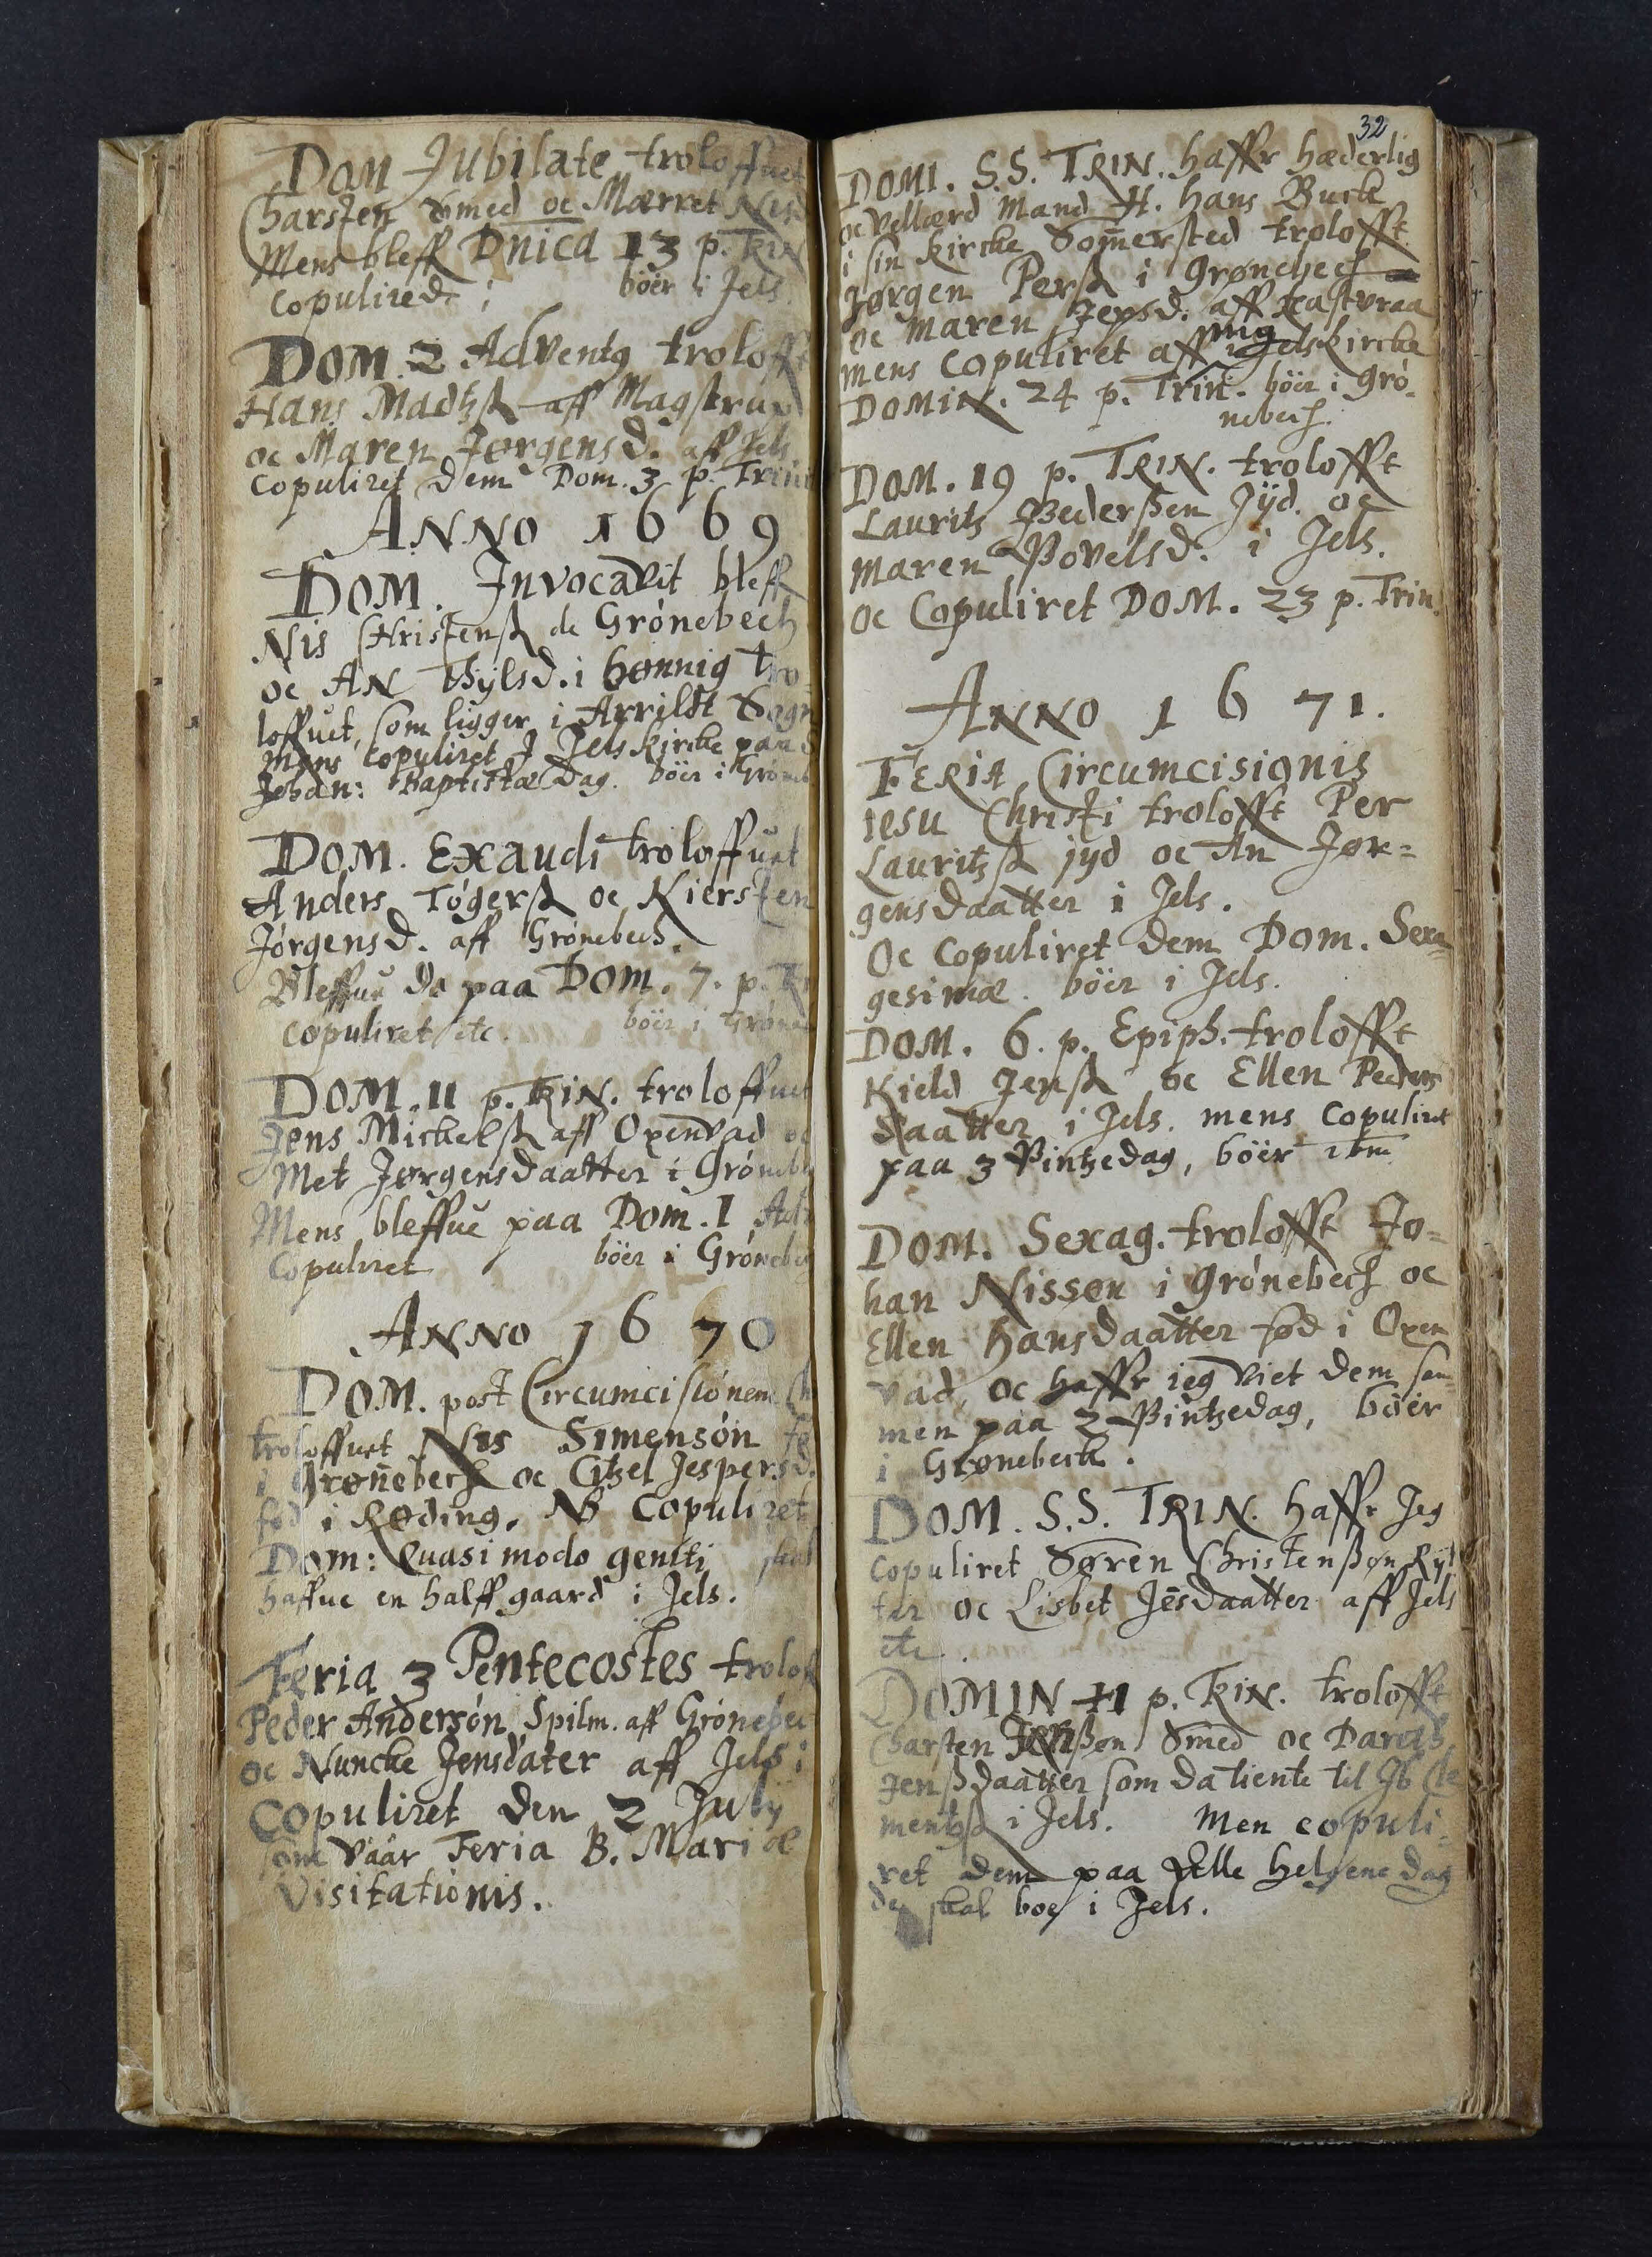DOM Jubilate troloffuet
Charsten Smed oc Marret Nisd.
Mens bleff Dnica 13 p. TRIN.
copulirede: boer i Jels.
DOM 2. Adventq trolofft
Hans Madtzs aff Magstrup
oc Maren Jørgensd. aff Jels.
Copuliret dem Dom. 3 p. Trinit
ANNO 1669
DOM. Invocavit bleff
Nis Christens de Grønnebech
oc An Thijlsd. I Hønnig tro¬
loffuet, som ligger i Arrildt Sogn
mens copuliret J Jels Kircke paa S
Johan: Baptista Dag. boer i Grøneb
DOM. Exaudi troloffuet
Anders Tøgers oc Kiersten
Jørgensd. aff Grønebech.
Bleffue de paa Dom. 7. p. Tr
copuliret etc. boer i Grøneb
DOM. II p. TRIN. troloffuet
Jens Mickels aff Oxenvad oc
Met Jørgens Daatter i Grønbe
Mens bleffue paa Dom. I Ad 
copuliret.
boer i Grønebe 
ANNO 1670
DOM. post Circumisionen Ch 
troloffuet NIS Simensøn
i Grønnebech oc Citzel Jespersd.
Fød i Røding. NB copuliret
Dom: Cuasi modo geniti skal
haffue en halff gaard i Jels.
Feria 3 Pentecostes trolof 
Peder Andersøn Spilm. aff Grønebec 
oc Nuncke Jensdater aff Jels:
copuliret den 2 Julij
som vaar Feria B. Maria
Visitationis.
32
DOMI. S.S. TRIN. Haffe Hæderlig
oc Vellærd Mand H. Hans Buck
i sin Kircke Sommersted trolofft
Jørgen Pers i Grønnebech
oc Maren Jepsd. aff Kastvraa
mig
mens copuliret aff i Jels Kircke
DOMIN. 24 p. Trin. boer i Grø¬
nebech.
DOM. 19 p. TRIN. trolofft
Lauritz Pederssen Jyd oc
Maren Povelsd. i Jels
oc copuliret DOM. 23 p. Trin.
ANNO 1671.
FERIA Circumcignis
iesu Christi trolofft Per
Lauritzs jyd oc An Jør¬
gens Daatter i Jels.
Oc Copuliret dem Dom. Sexa¬
gesima boer I Jels.
DOM. 6. P. Epiph. trolofft
Kield Jens oc Ellen Peders
daatter i Jels. Mens copuliret
paa 3 Pintzesag , boer ibm
DOM Sexag. trolofft Jo¬
han Nissen i Grønebech oc
Ellen Hansdaatter fød i Oxen
vad oc haffe ieg viet dem sam¬
men paa 2. Pintzedag, boer
i Grønebeck.
Dom S.S. TrRIN. haffe Jeg
copuliret Søren Christenssøn Ryt
ter oc Lisbet Jesdaatter aff Jels
etc.
DOMIN † 1 p. TRIN. trolofft
Charsten Jenssøn Smed oc Daras##
Jenssdaatter som da tiente til Ib Cle
mentzs i Jels. Men copuli¬
ret dem paa Alle Helgens Dag
de skal boe i Jels.
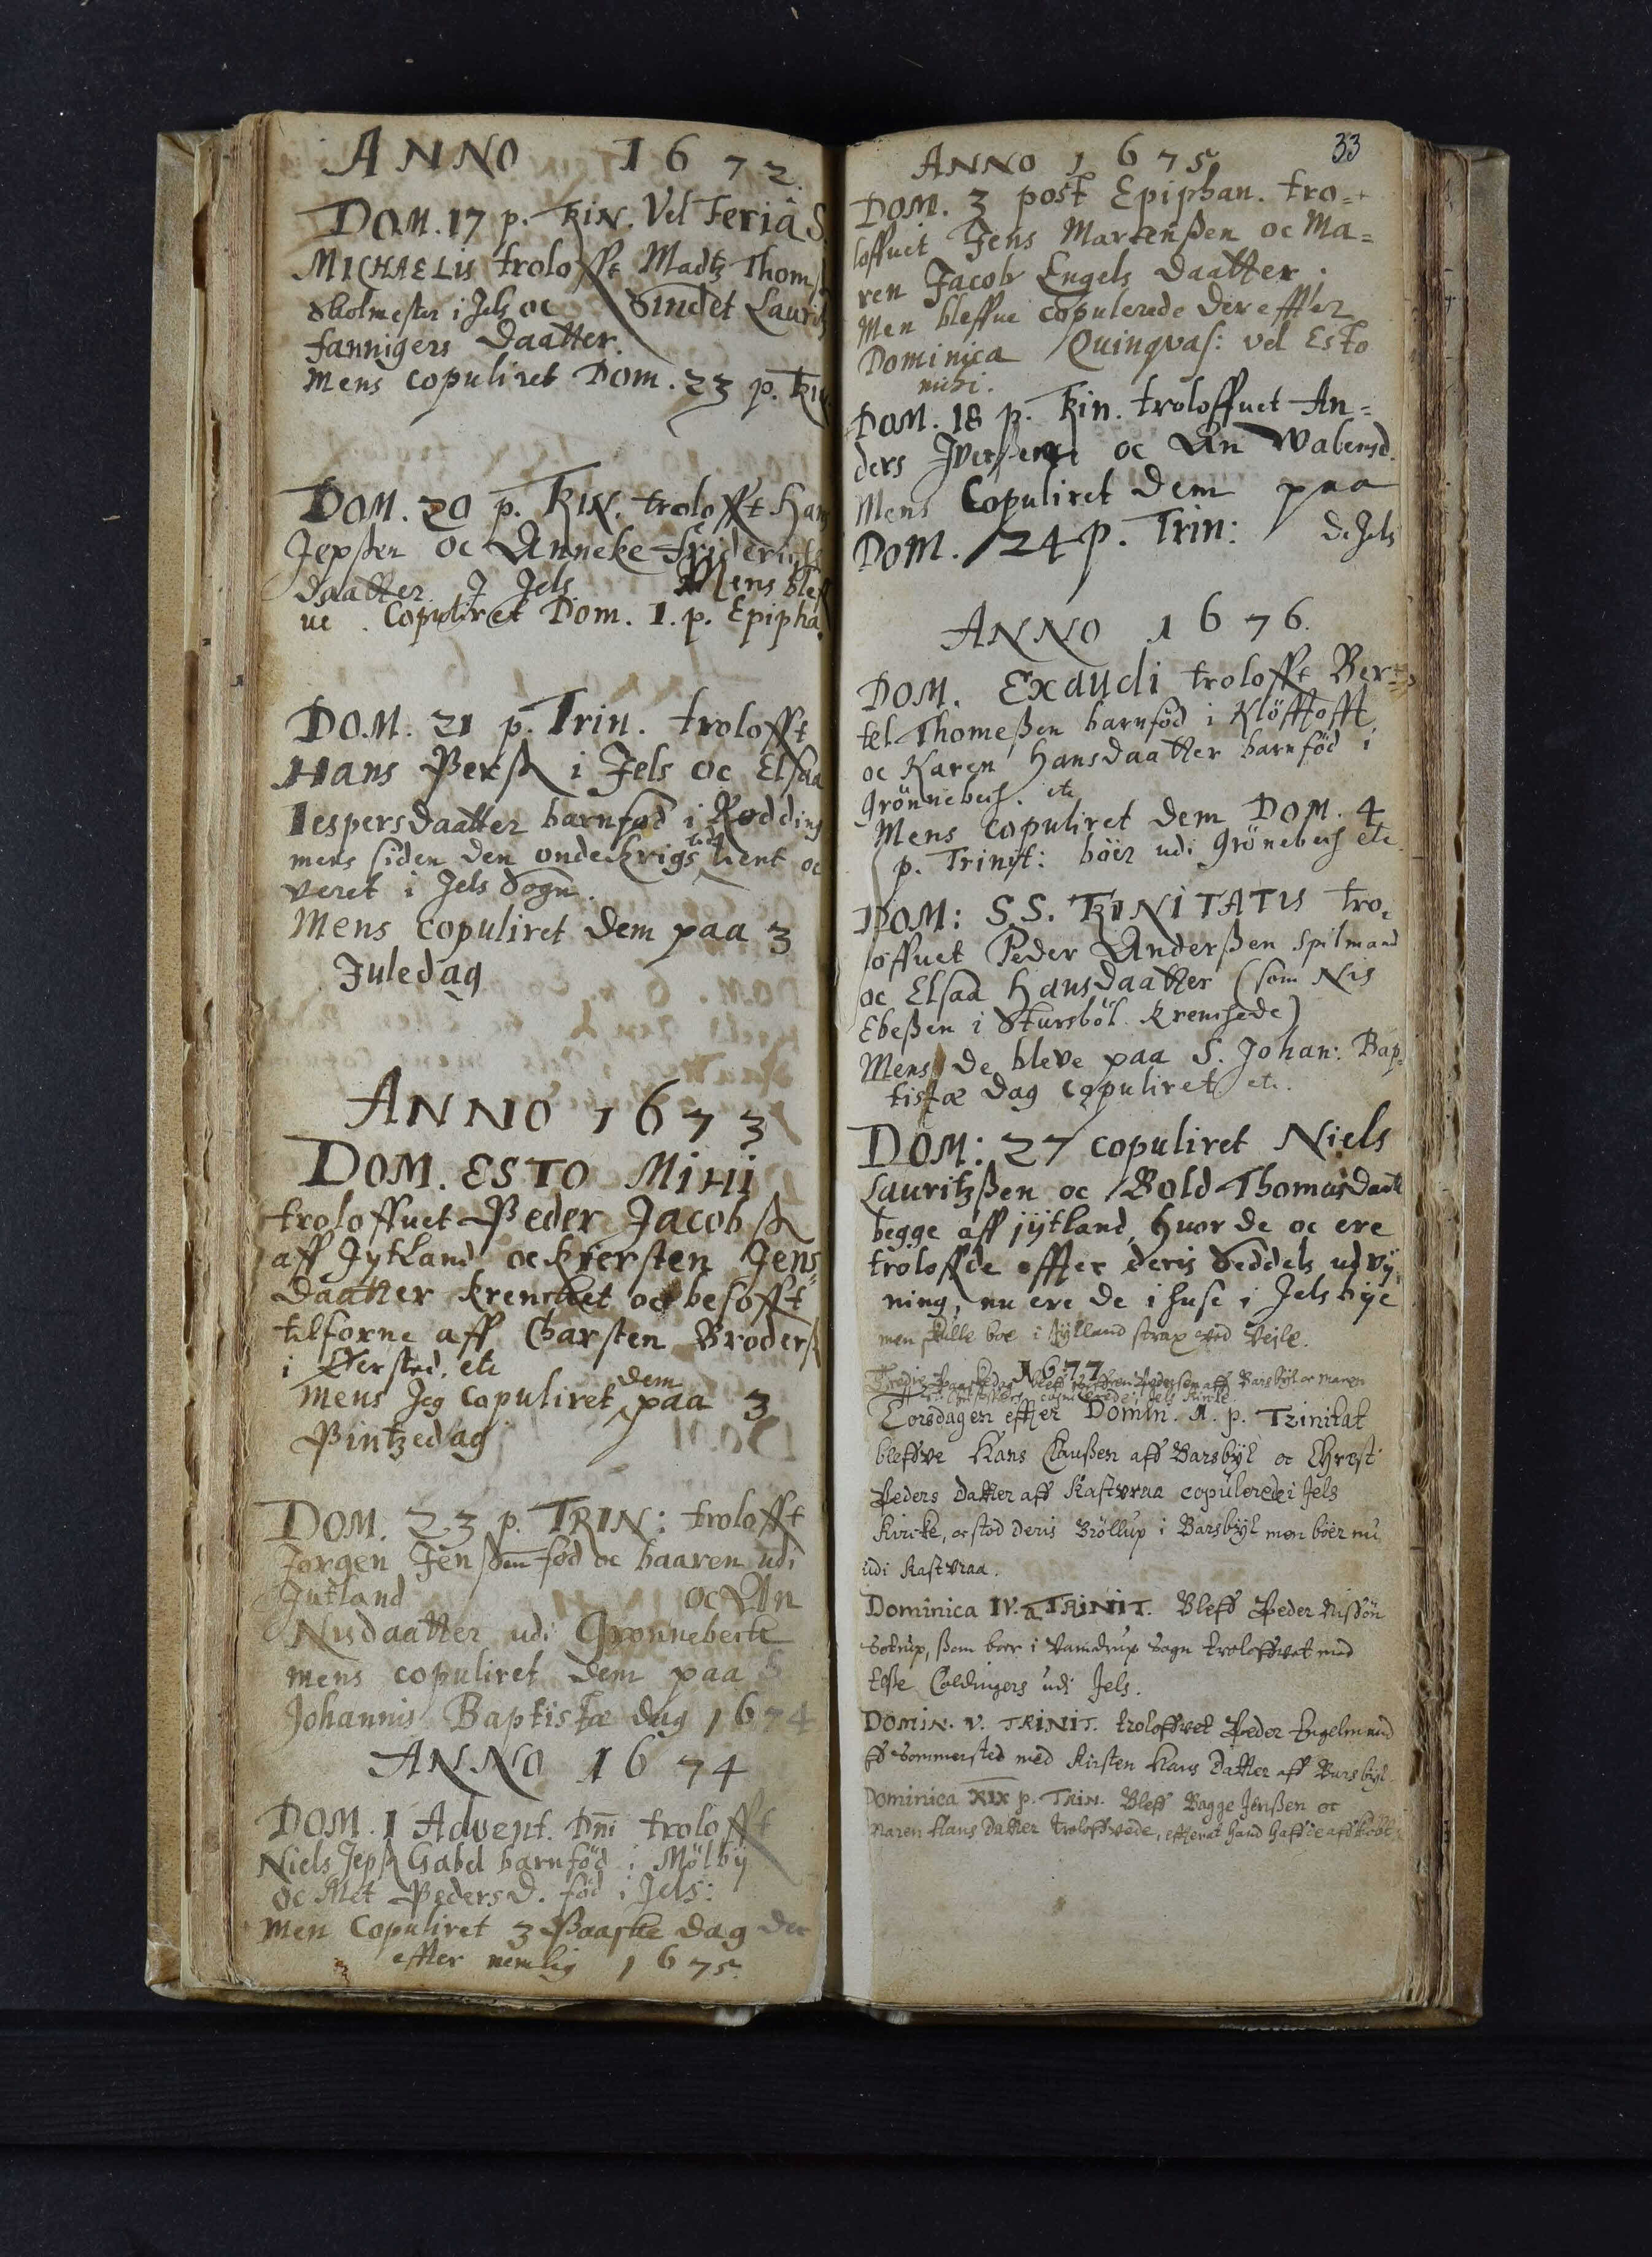ANNO 1672.
DOM. 17 p. TRIN. Vel Feria S
Michaelis trolofft Madtz Thoms 
Skolmester i Jels oc Sindet Lauritz
Fannigers Daatter.
Mens copuliret Dom. 23 p. Trin
DOM. 20 p. TRIN. trolofft Hans
Jepsen oc Anneke Friderichs
Daatter J Jels. Mens bleff
ue copuliret Dom. 1. p. Epipha
DOM. 21 p. Trin. trolofft
Hans Pers i Jels oc Elsaa
Iespers Daatter barnfød i Rødding
tid
mens siden den onde Krigs tient oc 
veret i Jels Sogn
Mens copuliret dem paa 3
Juledag
ANNO 1673.
DOM. ESTO MIHI
troloffuet Peder Jacobs
aff Jylland oc Kiersten Jens¬
Daatter krencket oc besofft
tilforne aff Charsten Broders
i Øersted etc.
dem
Mens Jeg copuliret paa 3
Pintzedag
DOM. 23 p. TRIN: trolofft
Jørgen Jensen fød oc baaren udi
Jutland
oc An
Nisdaatter udi Grønnebeck
mens copuliret dem paa S.
Johannis Baptista dag 1674
ANNO 1674
DOM. I Advent Dni trolofft
Niels Jeps Gabel barnfød i Mølby
oc Met Pedersd. fød i Jels:
men copuliret 3 Paaske Dag der
effter nemlig 1675.
33
ANNO 1675
DOM. 3 post Epiphan. Tro¬
loffuit Jens Martensen oc Ma¬
ren Jacob Engels Daatter.
Men bleffue copulerede der effter
Dominica Cuingvas: vel Esto
nuhi.
DOM. 18 p. TRIN. troloffuet An¬
ders Iversen oc An Wabensd.
Mens copuliret dem paa
Dom. 24 p. Trin: de Jels.
ANNO 1676
DOM. Exaudi trolofft Ber¬
tel Thomesen barnfød i Kløfftofft
oc Karen Hansdaatter barnfød i
Grønnebeck. etc.
Mens copuliret dem DOM. 4
p. Trinit: Boer udi Grønnebeck etc.
DOM: S. S. TRINITATIS tro¬
loffuet Peder Andersen Spilmand 
oc Elsaa Hansdaatter (som Nis 
Ebesen i Stursbøl krenchede)
Mens de bleve paa S. Johan: Bap¬
tista Dag copuliret etc.
DOM: 27 copuliret Niels
Lauritzsen oc Bold Thomasdaatt
begge aff Jytland, huor de oc ere
troloffde ##ffer deris Seddels udvy¬
ning, nu ere de i huse i Jels bye
men skulle boe i Jylland strax ved Vejle.
1677
Tredje Paaskedag bleff ## Pedersen aff Barsbyl oc Maren
S## Christoffers copulerede i Jels Kircke.
Torsdagen effter Domin. 1. p. Trinitat
bleffve Hans Clausen aff Barsbyl oc Chrest
Peders Datter aff Kastvraa copulerede i Jels
Kircke, oc stod deris Brøllup i Barsbyl men boer nu
udi Kastvraa.
Dominica IV. a. TRINIT. Bleff Peder Nissøn
Sotrup, som boer i Vamdrup Sogn troloffvet med
Elsse Coldingers udi Jels.
DOMIN. V. Trinit. troloffvet Peder Engelmand
 Sommersted med Kirsten Hans Datter aff Barsbyl
Dominica XIX p. Trin. Bleff Bagge Jensen oc
Maren Hans Datter troloffvede, efterat hand haffde aff